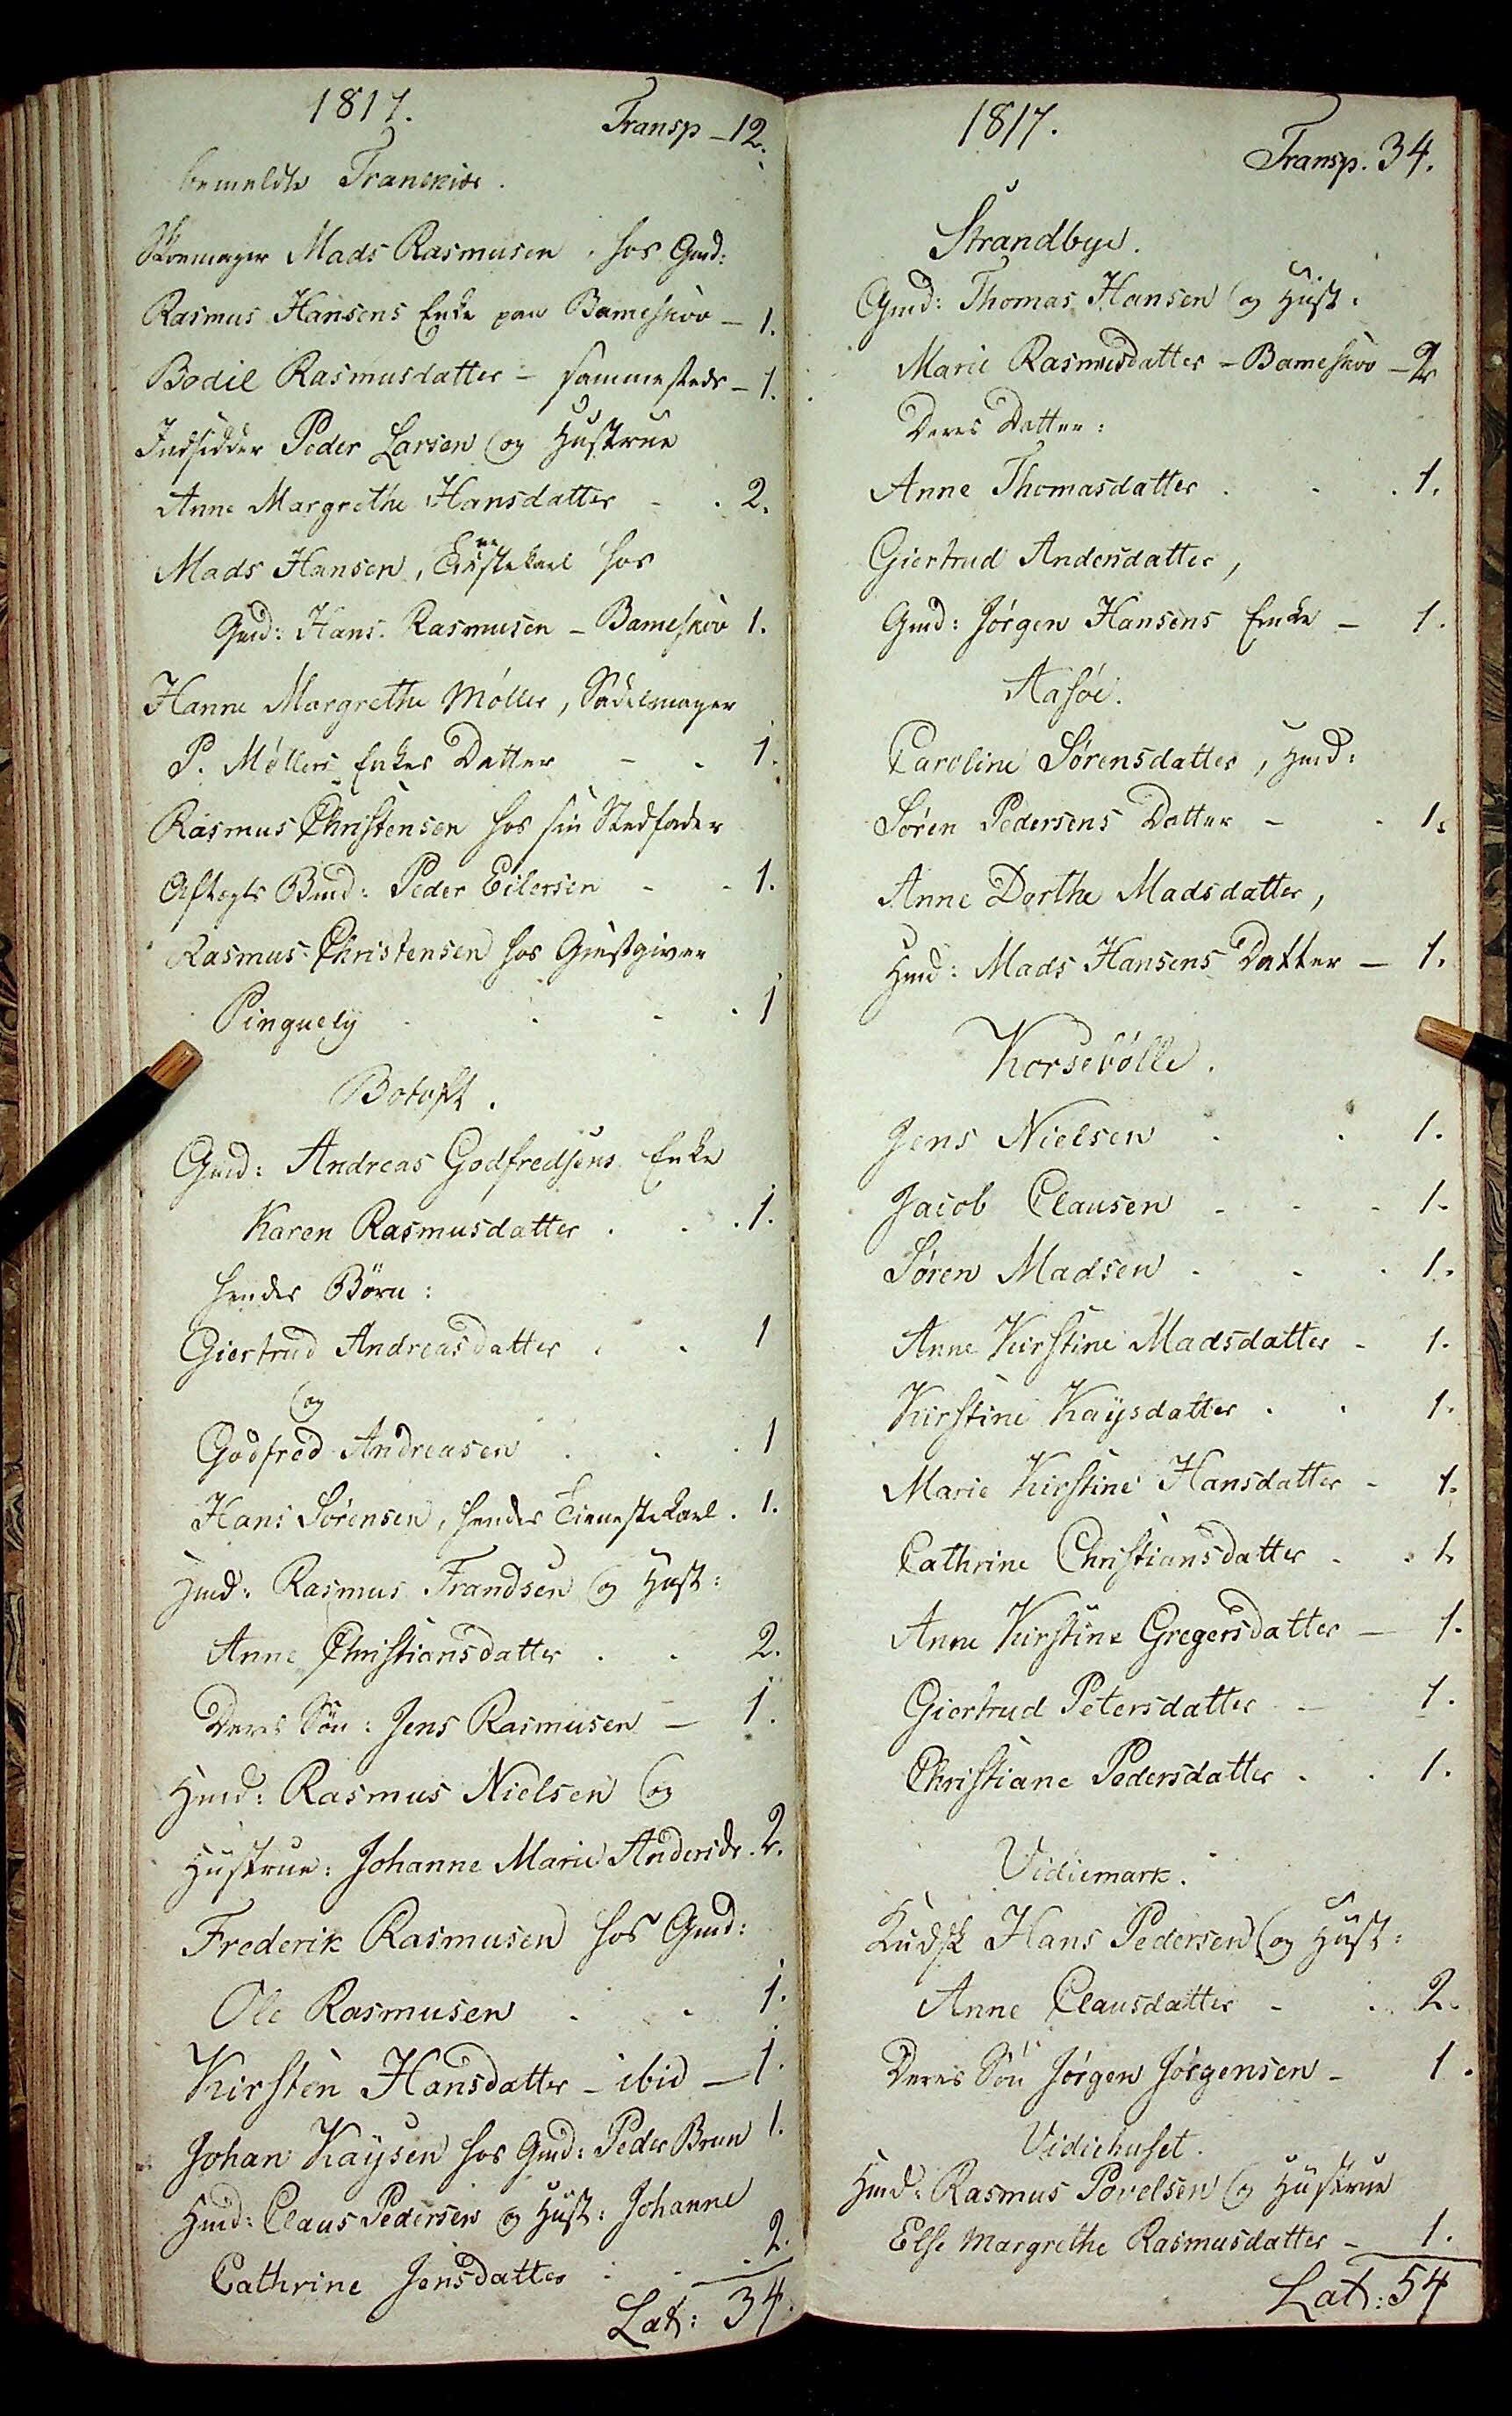1817
Transp - 12.
bemeldte Tranekiær.
Skoemager Mads Rasmusen hos Grd:
Rasmus Hansens Enke paa Bameskov - 1.
Bodil Rasmusdatter - sammesteds - 1.
Indsidder Peder Larsen og Hustrue
Anne Margrethe Hansdatter . . 2.
ne
Mads Hansen, Tiestekarl hos
Gmd: Hans Rasmusen - Bameskov 1.
Hanne Margrethe Møller, Sadelmager
P. Møllers Enkes Datter - . 1.
Rasmus Christensen hos sin Stedfader
Aftægts Bmd: Peder Eilersen . . 1.
Rasmus Christensen hos Giestgiver
Pinquely . . . . 1
Botoft.
Gmd: Andreas Godfredsens Enke
Karen Rasmusdatter . . . 1.
hendes Børn:
Giertrud Andreasdatter . . 1
og
Godfred Andreasen . . . 1
Hans Sørensen, hendes Tienestekarl . 1.
Hmd: Rasmus Frandsen og Hust:
Anne Christiansdatter . . 2.
Deres Søn: Jens Rasmusen - 1.
Hmd: Rasmus Nielsen og
Hustrue: Johanne Marie Andersdr . 2.
Frederik Rasmusen hos Gmd:
Ole Rasmusen . . 1.
Kirsten Hansdatter - ibid - 1
Johan Kaysen hos Gmd: Peder Brun 1
Hmd: Claus Pedersen og Hust: Johanne
Cathrine Jensdatter . . . 2
Lat: 34
1817
Transp. 34.
Srrandbye.
Gmd: Thomas Hansen og Hust:
Marie Rasmusdatter - Bameskov - 2
Dens Datter:
Anne Thomasdatter . . . 1.
Giertrud Andersdatter
Gud: Jørgen Hansens Enke - 1.
Aasøe.
Caroline Sørensdatter, Hmd:
Søren Pedersens Datter - . 1.
Anne Dorthe Madsdatter,
Hmd: Mads Hansens Datter - 1.
Korsebølle.
Jens Nielsen . . 1.
Jacob Clausen . . . 1.
Søren Madsen . . . 1.
Anne Kirstine Madsdatter - 1.
Kirstine Kaysdatter . . 1.
Marie Kirstine Hansdatter . 1.
Cathrine Christiansdatter . . 1.
Anna Kirstine Gregersdatter - 1.
Giertrud Pedersdatter - 1.
Christiane Pedersdatter . . 1.
Vidiemark.
Kudsk Hans Pedersen og Hust:
Anne Clausdatter - . 2.
Deres Søn Jørgens Jørgensen - 1.
Vidiehuset.
Hmd: Rasmus Povelsen og Hustrue
Else Margrethe Rasmusdatter - 1.
Lat: 54
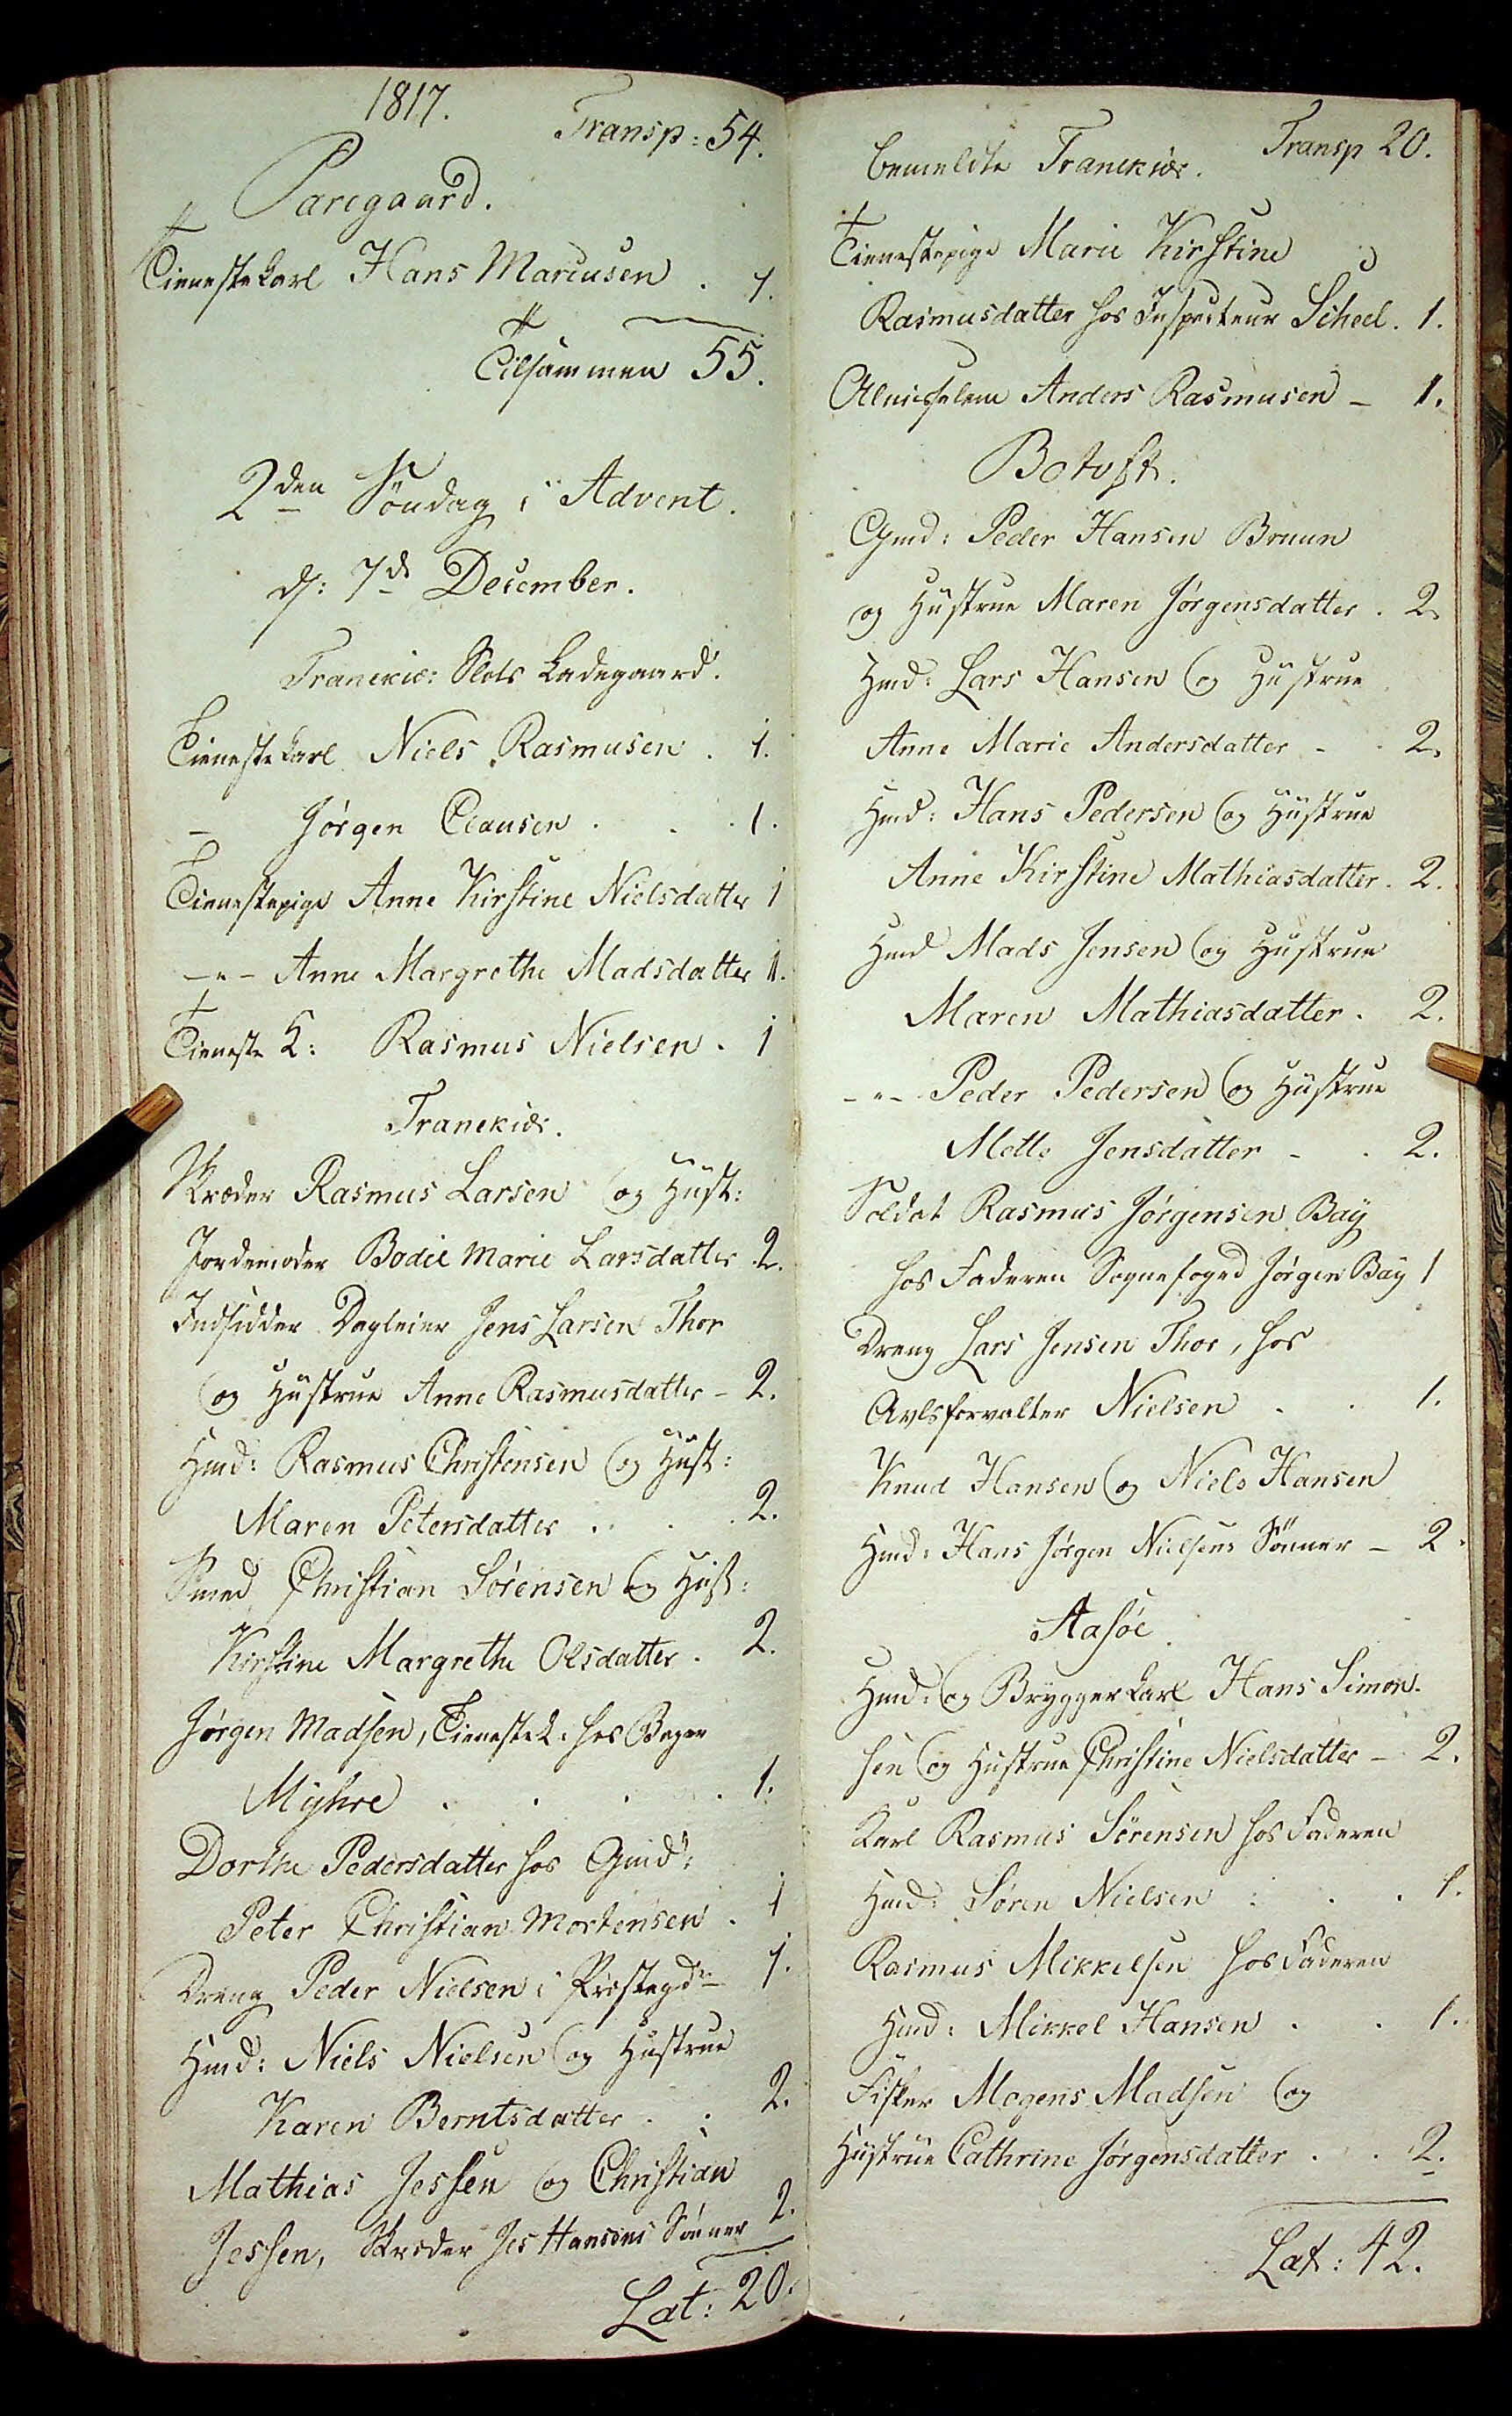1817.
Transp: 54.
Pæregaard.
Tienestekarl Hans Marcusen . 1.
Tilsammen 55.
2den Søndag i Advent.
d: 7de December.
Tranekiær Slots Ladegaard.
Tienestekarl Niels Rasmusen . 1.
- Jørgen Clausen . . . 1.
Tienestepige Anne Kirstine Nielsdatter 1
-"- Anne Margrethe Madsdatter 1.
Tieneste K: Rasmus Nielsen . 1
Tranekiær.
Skræder Rasmus Larsens og Hust:
Jordemoder Bodil Marie Larsdatter . 2.
Indsidder Dagleier Jens Larsens Thor
og Hustrue Anne Rasmusdatter - 2.
Hmd: Rasmus Christensen og Hust:
Maren Petersdatter . . . . 2.
Smed Christian Sørensen og Hust:
Kirstine Margrethe Olsdatter . 2.
Jørgen Madsen, Tienested: hos Bager
Myhre . . . . 1.
Dorthe Pedersdatter hos Gmd:
Peter Christian Mortensen . 1
Dreng Peder Nielsen i Præstegdr 1
Hmd: Niels Nielsen og Hustrue
Karen Berntsdatter . . 2.
Mathias Jessen og Christian
Jessen, Skreder Jes Hansens Sønner 2.
Lat: 20
Transp 20.
bemeldte Tranekiær.
Tienestepige Marie Kirstine
Rasmusdatter hos Inspecteur Scheel . 1.
Almisselem Anders Rasmusen - 1.
Botoft.
Gmd: Peder Hansens Bruun
og Hustrue Maren Jørgensdatter . 2.
Hmd: Lars Hansen og Hustrue
Anne Marie Andersdatter . . 2.
Hmd: Hans Pedersen og Hustrue
Anne Kirstine Mathiasdatter . 2.
Hmd Mads Jensen og Hustrue
Maren Mathiasdatter . 2.
-"- Peder Pedersen og Hustrue
Mette Jensdatter - . 2.
Soldat Rasmus Jørgensen Bay
hos Faderen Sognefoged Jørgen Day 1
Dreng Jens Jensen Thor, hos
Avlsforvalter Nielsen . . 1.
Knud Hansen og Niels Hansen
Hmd: Hans Jørgen Nielsens Sønner - 2
Aasøe
Hmd: og Bryggerkarl Hans Simon¬
sen og Hustrue Christine Nielsdatter - 2.
Karl Rasmus Sørensen hos Faderen
Hmd: Søren Nielsen . . . 1.
Rasmus Mikkelsen hos Faderen
Hmd: Mikkel Hansen . . 1.
Fisker Mogens Madsen og
Hustrue, Cathrine Jørgensdatter . . 2.
Lat: 42.